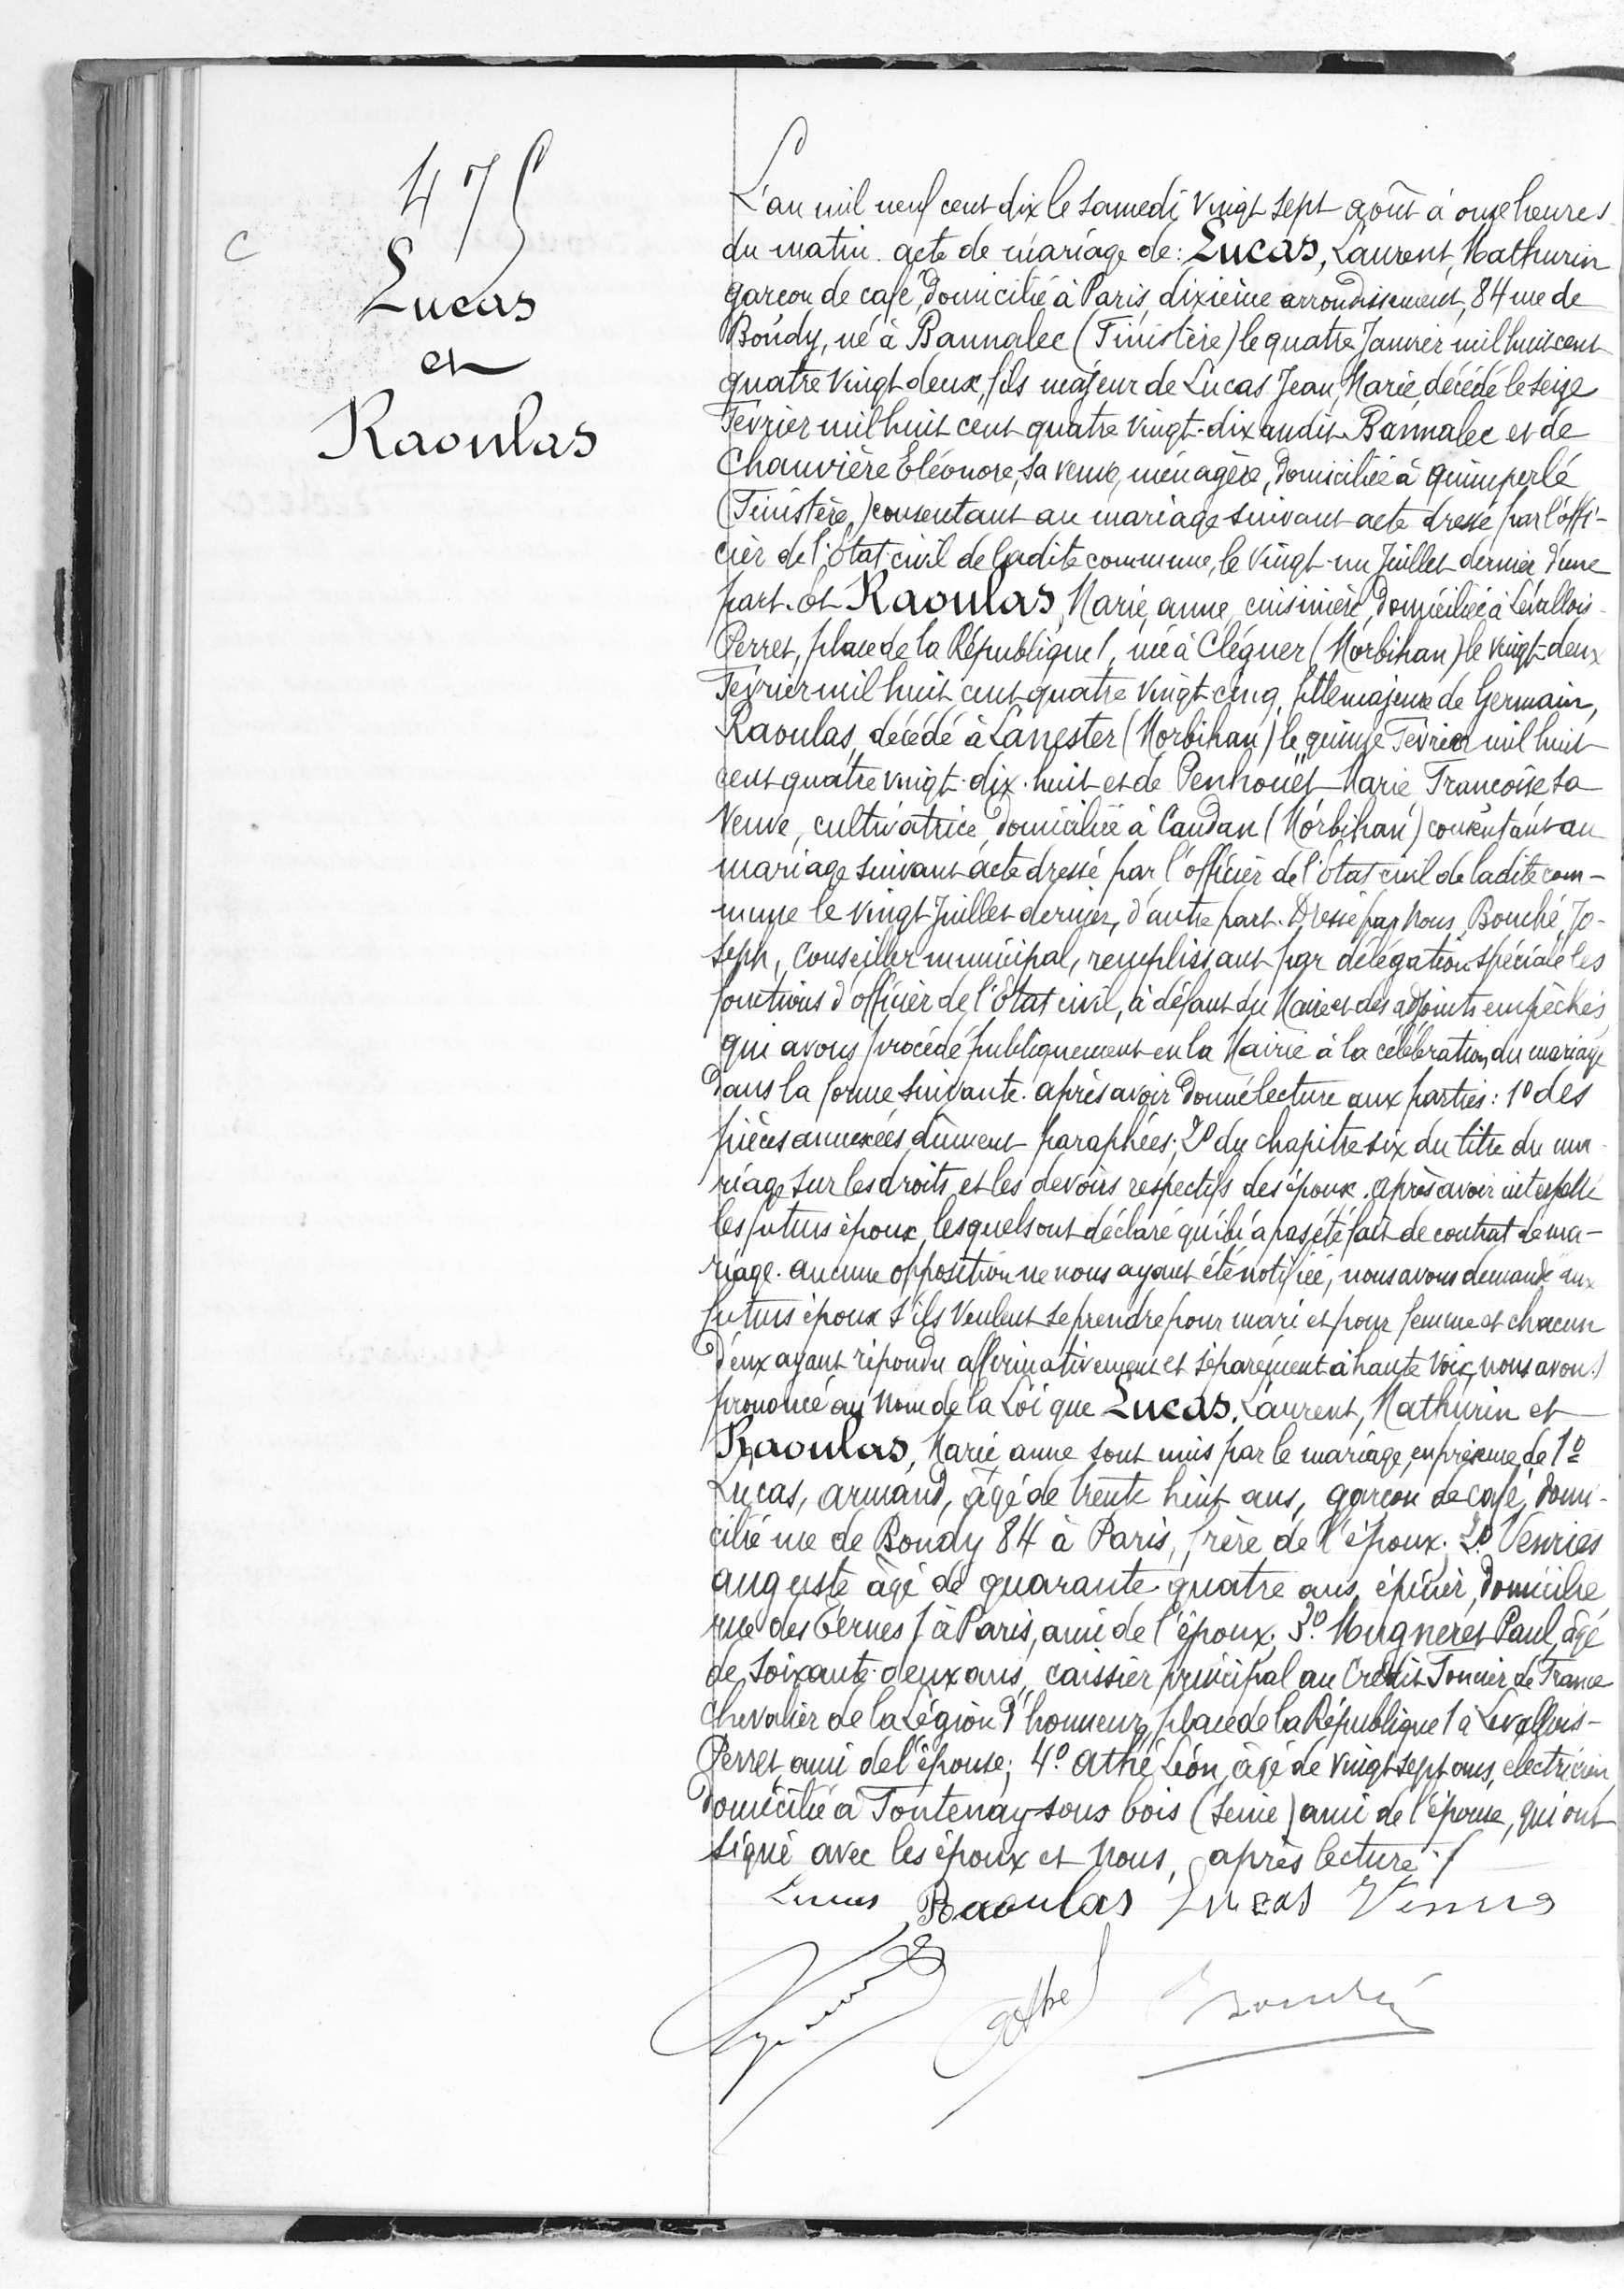475
Lucas
et
Raonlas
L'an mil neuf cent dix le samedi vingt sept août à onze heures
du matin acte de mariage de: Lucas, Laurent, Mathurin
Garçon de cafe, domicilié à Paris, dixième arrondissement, 84 rue de
bondy, né à Baunalec (Finistère) le quatre Janvier mil huit cent
quatre vingt deux, fils majeur de Lucas Jean, Marie, décédé le seize
Février mil huit cent quatre vingt-dix audit Bannalec et de
Chauvière Eléonore, sa veuve, ménagère, Domiciliée à quimperlé
(Finistère) consentant au mariage suivant acte dressé par l'offi-
cier de l'Etat Civil de ladite commune, le Vingt-un Juillet dernier d'une
part. et Raoulas, Marie, anne, cuisinière, domiciliée à Levallois-
Perret, Place de la République, née à Cléguer (Morbihan) le vingt-deux
Fevrier mil huit cent quatre vingt-cinq, fille majeure de Germain,
Raoulas, décédé à Lanester (Morbihan) le quinze Fevrier mil huit
cent quatre-vingt-dix. huit et de Penhouët Marie Francône sa
Veuve, cultivatrice, domiciliée à Caudan (Morbihan) consentante au
mariage suivant acte dressé par l'officier de l'Etat civil de ladite com-
mune le vingt Juillet dernier, d'autre part. Dressé par Nous, Bouché, Jo-
seph, conseiller municipal, remplissant par délégation spéciale les
fonctions d'officier de l'Etat civil, à défaut du Maire et des adjoints empéchés
qui avons procédé publiquement en la Mairie à la célébration du mariage
dans la forme suivante. après avoir donné lecture aux parties: 1° des
pièces annexées dûment paraphées; 2° du chapitre six du titre du ma-
riage sur les droits et les devoirs respectifs des époux. Après avoir interpellé
les futurs époux, lesquels ont déclaré qu'ils n'a pas été fait de contrat de ma-
riage. aucune opposition ne nous ayant été notifiée, nous avons demandé aux
futurs époux s'ils veulent se prendre pour mari et pour femme et chacun
d'eux ayant répondu affirmativement et séparément à haute voix, nous avons
prononcé au Nom de la Loi que Lucas, Laurent, Mathurin et
Raoulas, Marie anne sont unis par le mariage, en présence de 1°
Lucas, armand, agé de trente huit ans, garçon de café domi-
cilié rue de Bondy 84 à Paris, frère de l'époux,2° Venries
auguste âgé de quarante-quatre ans épicier, domicilié
rue des Ternes, 1 à Paris, ami de l'époux. 3° Mugneres Paul, agé
de soixante-deux ans, caissier municipal au Credit Foncier de France
Chevalier de la Légion d'honneur, place de la République 1 à Levallois-
Perret ami de l'épouse; 4° Athé Léon agé de vingt-sept ans, électricien,
domicilié à Fontenay-sous bois (Seine) ami de l'épouse, qui ont
signé avec les époux et Nous, après lecture ./.
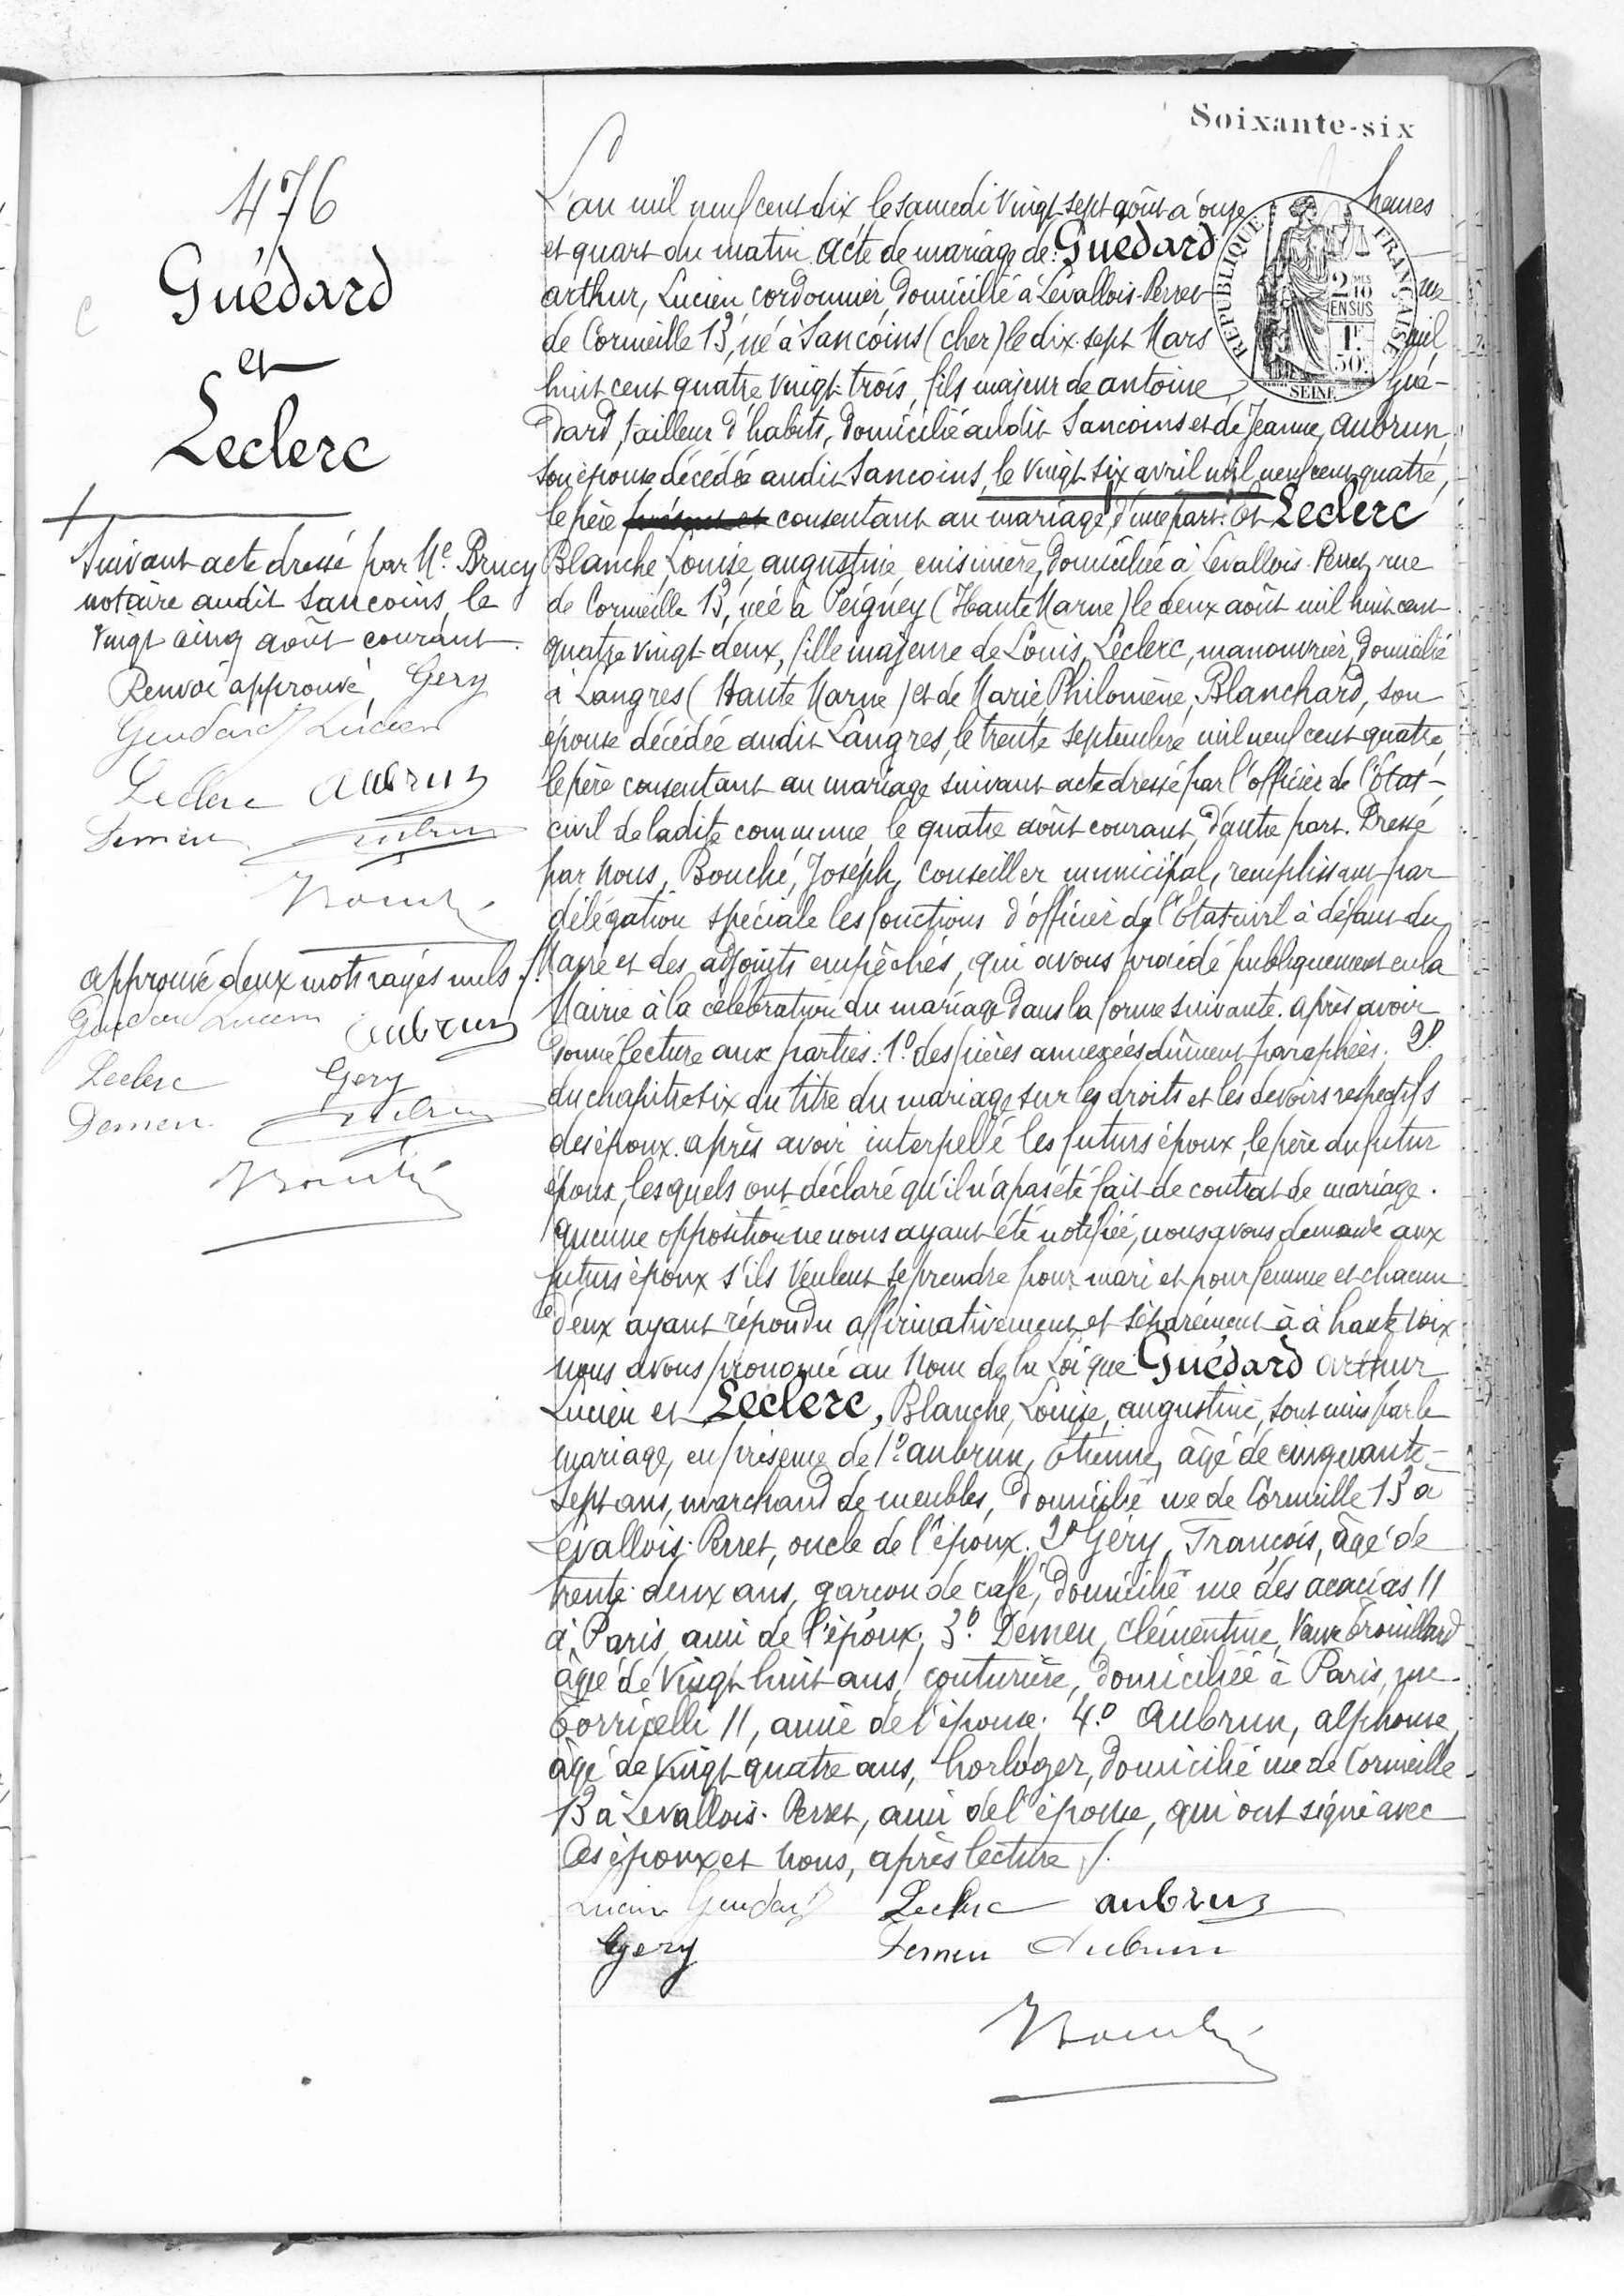476
Guédard
et
Leclerc
L'an mil neuf cent dix le samedi vingt-sept août à onze heures
et quart du matin acte de mariage de: Guédard
arthur, Lucien, cordonnier, domicilié à Levallois-Perret rue
de Cormeille 13, né à à Sancoins (cher) le dix-sept Mars mil
huit cent quatre vingt trois, fils majeur de antoine Gué-
dard, tailleur d'habits, domicilié audit Sancois et de Jeanne, Aubrun
son épouse décédée audit Sancoins, le vingt-six avril mil neuf cent quatre,
le père présent et consentant au mariage, d'une part. Et Leclerc
Blanche, Louise, augustine, cuisinière, domiciliée à Levallois-Perret, rue
de Cormeille, 13, née à la Peigney (Haute Marne) le deux août mil huit cent
quatre vingt-deux, fille majeure de Louis Leclerc, manoeuvrier domicilié
à Langres (Haute Marne) et de Marie Philomène, Blanchard, son
épouse décédée audit Langres, le trente septembre mil neuf cent quatre,
le père consentant au mariage suivant acte dressé par l'officier de l'Etat-
civil de la dite commune le quatre août courant, d'autre part. Dressé
par Nous. Bouché, Joseph, conseiller municipal, remplissant par
délégation spéciale les fonctions d'officier de l'Etat civil à défau du
Maire et des adjoints empèchés, qui avons procédé publiquement en la
Mairie à la célébration du mariage dans la forme suivant après avoir
donné lecture aux parties. 1° des pièces anneyées dûment paraphées. 2°
du chapitre six du titre du mariage sur les droits et les devoirs respectifs
des époux. après avoir interpellé les futurs époux, le père du futur
époux, lesquels ont déclaré qu'il n'a pas été fait de contrat de mariage.
aucune opposition ne nous ayant été notifiée, nous avons demandé aux
futurs époux, s'ils veulent se prendre pour mari et pour femme et chacun
d'eux ayant répondu affirmativement et séparément à à haute voix
nous avons prononcé au Nom de la Loi que Guédard arthur
Lucien et Leclerc, Blanche, Louise, augustine, sont unis par le
mariage, en présence de 1° aubrun, Etienne, agé de cinquante-
sept ans, marchand de meubles, domicilié rue de Cormeille 13 à
Levallois-Perret, oncle de l'époux. 2° Géry François, âgé de
trente-deux ans, garçon de café, domicilié rue des acacias 11
à Paris, ami de l'époux; 3° Demeu, Clémentine, Veuve Trouillard
âgé de vingt huit ans, couturière, domiciliée à Paris, rue
Torricelli 11, amie de l'épouse. 4° Aubrun, alphonse
âgé de vingt-quatre ans, horloger, domicilié rue de Cormeille
13 à Levallois-Perret, ami de l'épouse, qui ont signé avec
les époux et nous, après lecture./.